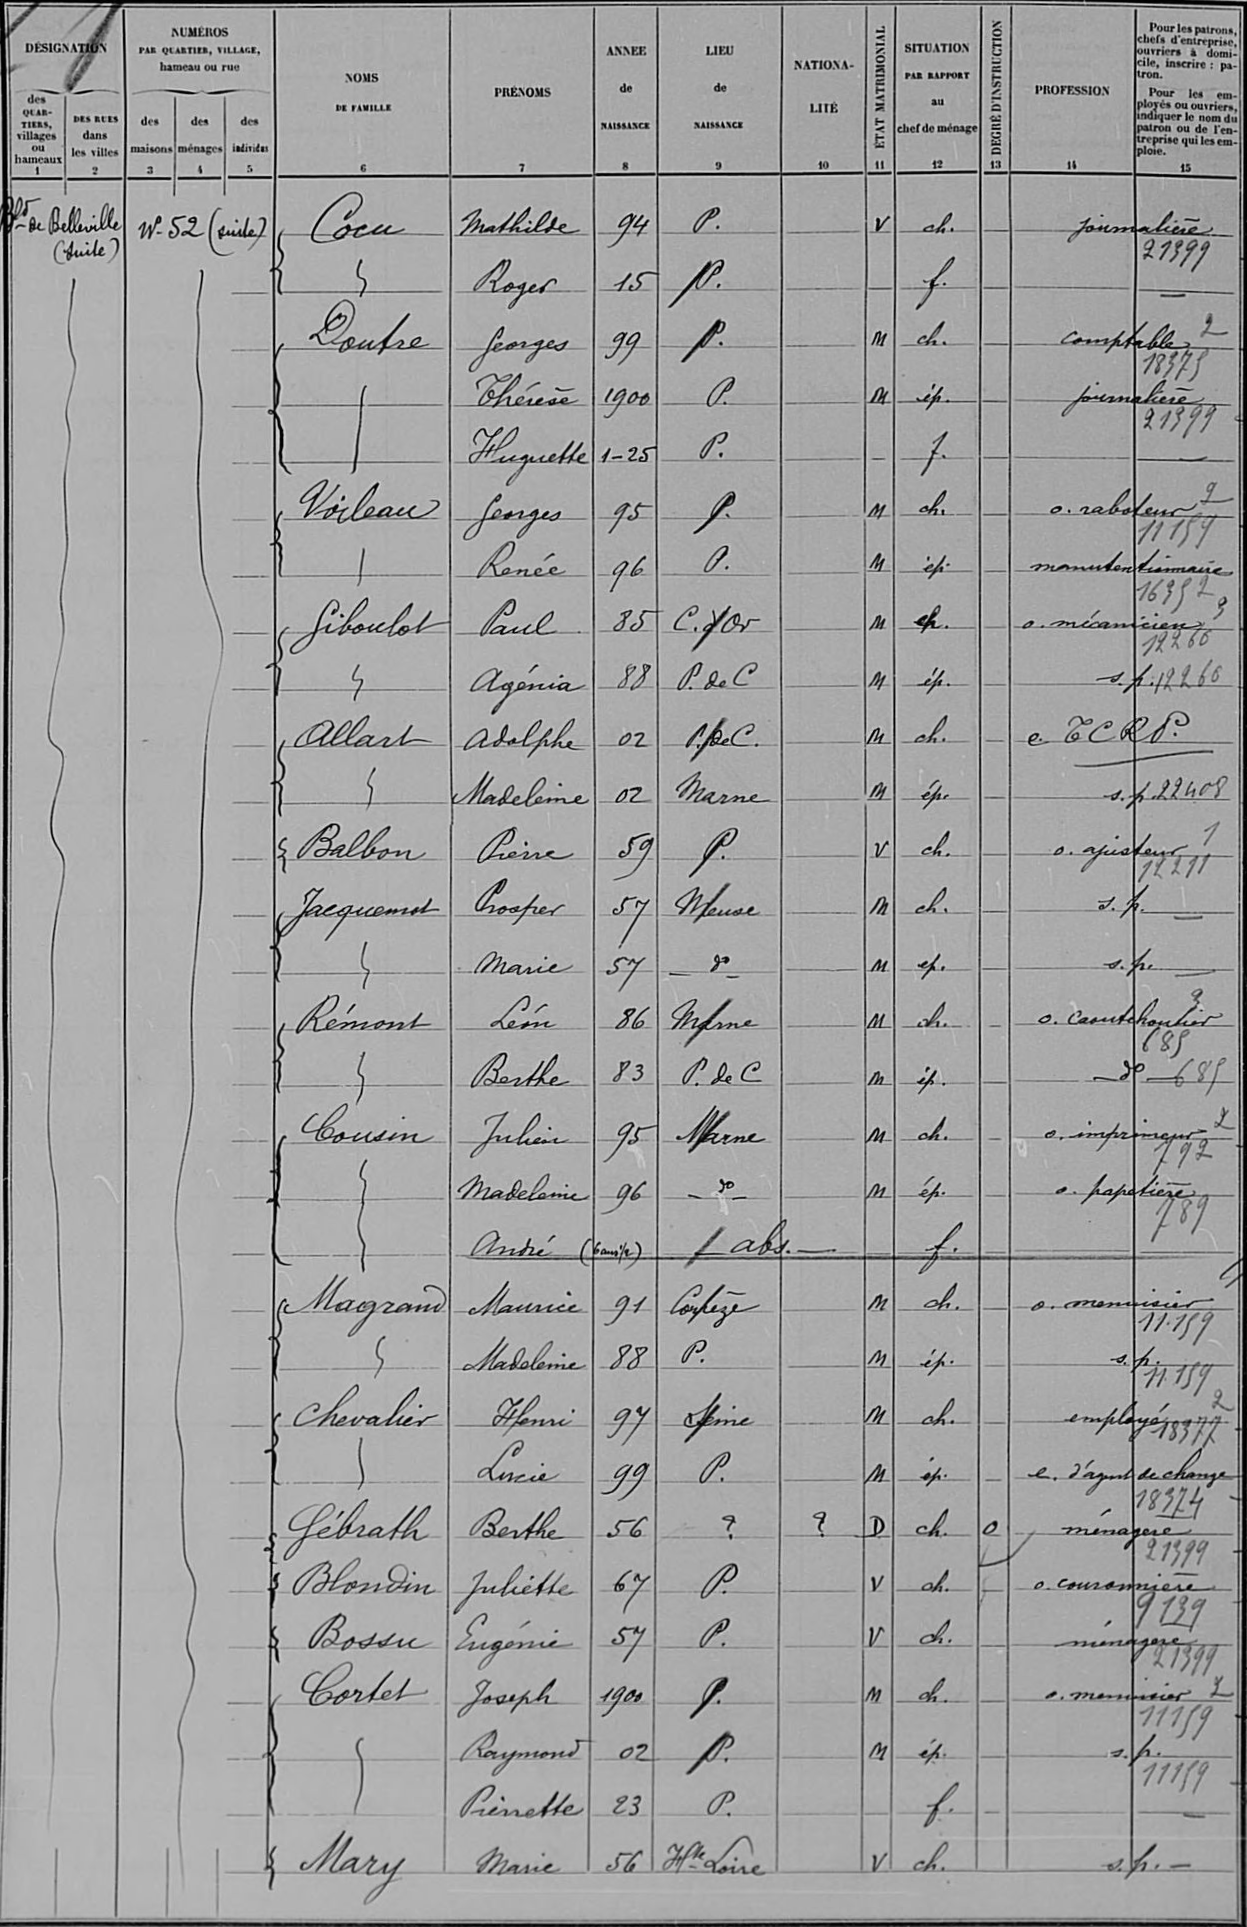Cocu/Mathilde/94/P /¤/V/ch /¤/journalière/¤
¤/Roger/15/P /¤/¤/f /¤/¤/¤/¤
Doutre/Georges/99/P /¤/M/ch /¤/comptable/¤
¤/Thérèse/1900/P /¤/M/ép /journalière/¤
¤/Huguette/1 25/P /¤/¤/f /¤/¤/¤/¤
Voileau/Georges/95/P /¤/M/ch /¤/o raboteur/¤
¤/Renée/96/P /¤/M/ép /¤/manutentionnaire/¤
Giboulot/Paul/85/C d'Or/¤/M/ep /¤/o mécanicien/¤
¤/Agénia/88/P de C/¤/M/ép /¤/s p /12260
Allart/Adolphe/02/P de C /¤/M/ch /¤/e C CRP /¤/¤
¤/madeleine/02/Marne/¤/M/ép /¤/s p /22408
Balbon/Pierre/59/P /¤/V/ch /¤/o ajusteur/122111
Jacquemot/Prosper/57/Meuse/¤/M/ch /¤/s p /¤/¤
¤/Marie/57/d°/¤/M/ep /¤/s p /¤/¤
Rémont/Léon/86/Marne/¤/M/ch /¤/o caoutchoutier/¤
¤/Berthe/83/P de C/¤/M/ép /¤/d°/685
Cousin/Julien/95/Marne/¤/M/ch /¤/o imprimeur/¤
¤/Madeleine/96/d°/¤/M/ép /¤/o papetière/¤
¤/André/(6 ans 1 2)/abs /¤/¤/f /¤/¤/¤/¤
Magrand/Maurice/91/Corrèze/¤/M/ch /¤/o menuisier/¤
¤/Madeleine/88/P /¤/M/ép /¤/s p /¤
Chevalier/Henri/97/Seine/¤/M/ch /¤/employé/¤
¤/lucie/99/P /¤/M/ép /¤/e d'agent de change/¤
Gébrath/Berthe/56/¤/¤/D/ch /O/ménagere/¤
Blondin/Juliette/67/P /¤/V/ch /¤/o couronnière/¤
Bossu/Eugénie/57/P /¤/V/ch /¤/ménagere/¤
Cortet/Joseph/1900/P /¤/M/ch /¤/o menuisier/¤
¤/Raymond/02/P /¤/M/ép /¤/s p /¤
¤/Pierrette/23/P /¤/¤/f /¤/¤/¤/¤
Mary/Marie/56/Hte Loire/¤/V/ch /¤/s p /¤/¤
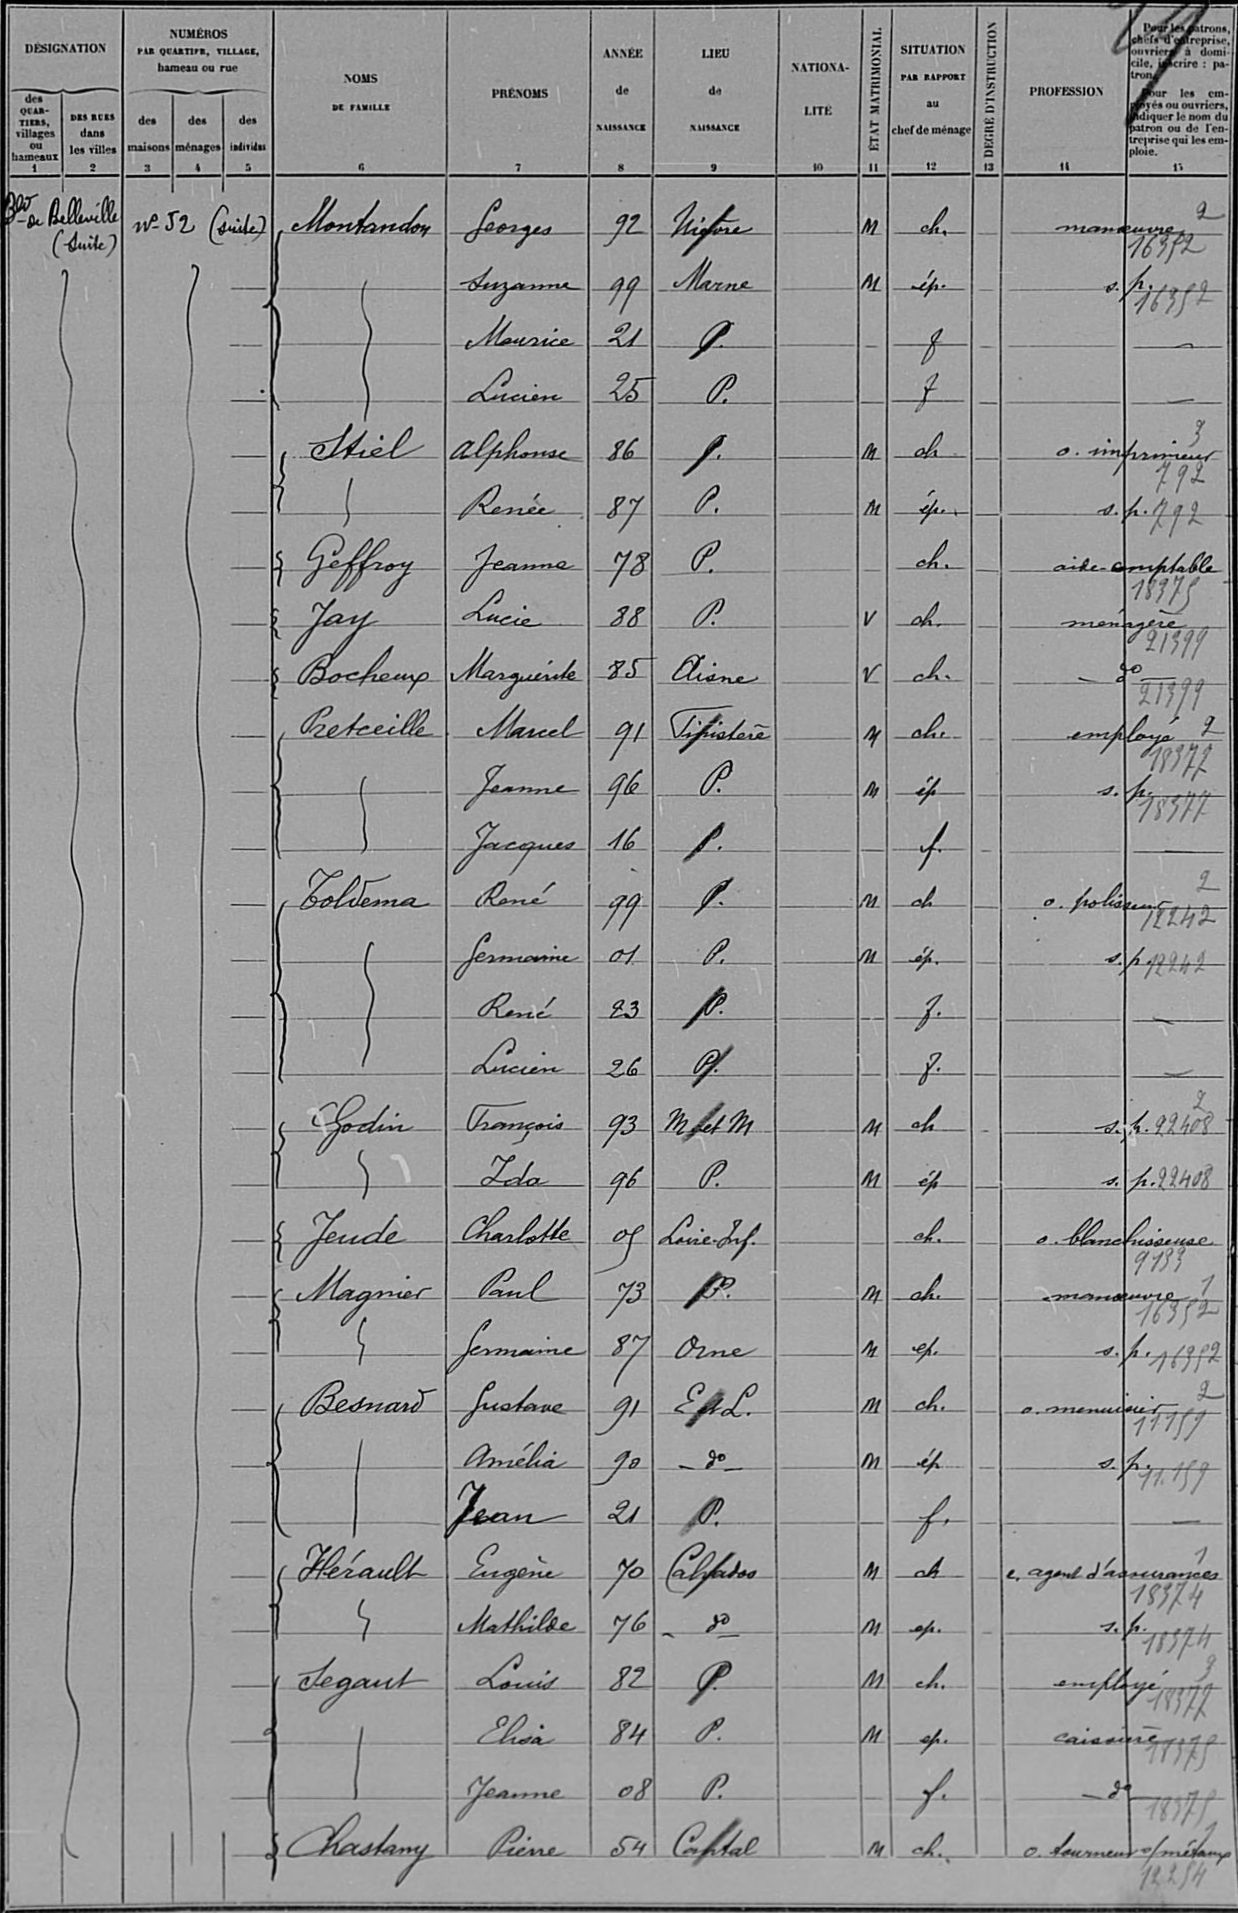Montandon/Georges/92/Nièvre/¤/M/ch /¤/manoeuvre/¤
¤/Suzanne/99/Marne/¤/M/ép /¤/s p /¤
¤/Maurice/21/P /¤/¤/f/¤/¤/¤/¤
¤/Lucien/25/P /¤/¤/f/¤/¤/¤/¤
Shiel/Alphonse/86/P /¤/M/ch/¤/o imprimeur/¤
¤/Renée/87/P /¤/M/ép /¤/s p /792
Geffroy/Jeanne/78/P /¤/¤/ch /¤/aide-comptable/¤
Jay/Lucie/88/P /¤/V/ch /¤/ménagere/¤
Bocheux/Marguerite/85/Aisne/¤/V/ch /¤/d°/¤/¤
Pretuille/Marcel/91/Finistère/¤/M/ch /¤/employé/¤
¤/Jeanne/96/P /¤/M/ép/¤/s p /¤
¤/Jacques/16/P /¤/¤/f /¤/¤/¤/¤
Boldena/René/99/P /¤/M/ch/¤/o polisseur/¤
¤/Germaine/01/P /¤/M/ép /¤/s p /12242
¤/René/23/P /¤/¤/f /¤/¤/¤/¤
¤/Lucien/26/P /¤/¤/f /¤/¤/¤/¤
Godin/François/93/M et M/¤/M/ch/¤/s p /22408 2
¤/ida/96/P /¤/M/ép/¤/s p /22408
Jeude/Charlotte/05/Loire-Inf/¤/¤/ch /¤/o blanchisseuse/¤
Magnier/Paul/73/P /¤/M/ch /manoeuvre/¤
¤/Germaine/87/Orne/¤/M/ep /¤/s p /16352
Besnard/Gustave/91/E et L /¤/M/ch /¤/o menuisier/¤
¤/Amélia/90/d°/¤/M/ép/¤/s p /¤
¤/Jean/21/P /¤/¤/f /¤/¤/¤/¤
Hérault/Eugène/70/Calvados/¤/M/ch/e agent d'assurances/¤
¤/Mathilde/76/d°/¤/M/ep /¤/s p /18374
Segaut/Louis/82/P /¤/M/ch /¤/employé/¤
¤/Elisa/84/P /¤/M/ep /¤/caissière/¤
¤/Jeanne/08/P /¤/¤/f /¤/d°/18375
Chastamy/Pierre/54/Cantal/¤/M/ch /¤/o tourneur s- métaux/¤
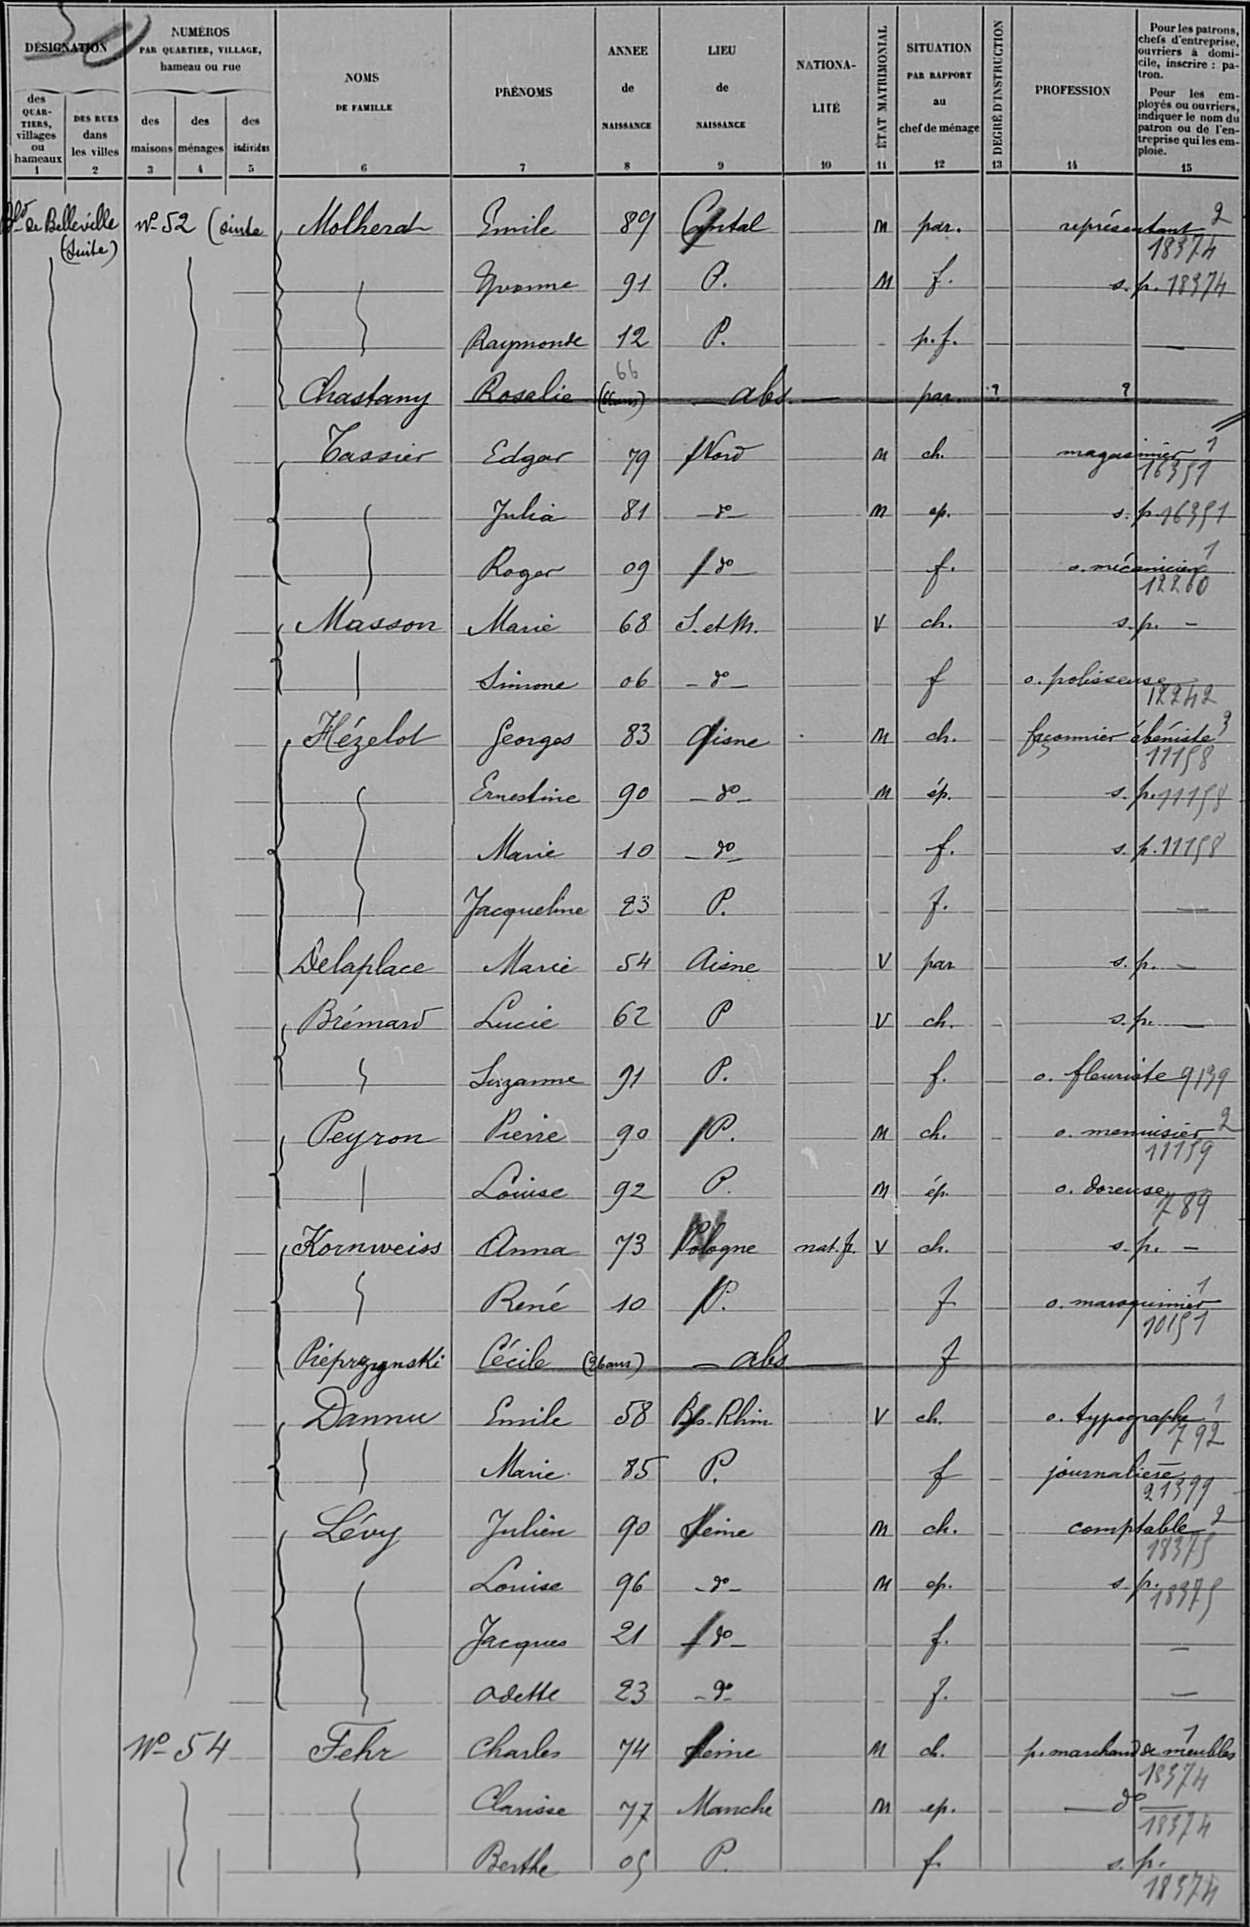Molherat/Emile/89/Cantal/¤/M/par /¤/représentant/¤
¤/Yvonne/91/P /¤/M/f /¤/s p /18374
¤/Raymonde/12/P /¤/¤/p f /¤/¤/¤/¤
Chastany/Roselie/(66 ans) 66/abs/¤/¤/par/¤/¤/¤/¤
Cassier/Edgar/79/Nord/¤/M/ch /¤/magasinier/¤
¤/Julia/81/d°/¤/M/ep /¤/s p/16351
¤/Roger/09/d°/¤/¤/f /¤/o mécanicien/12260 1
Masson/Marie/68/S et M /¤/V/ch /¤/s p /¤/¤
¤/Simone/06/d°/¤/¤/f/¤/o polisseuse/¤
Hézelot/Georges/83/Aisne/¤/M/ch /¤/façonnier ébéniste/¤
¤/ernestine/90/d°/¤/M/ép /¤/s p /11158
¤/Marie/10/d°/¤/¤/f /¤/s p /11158
¤/jacqueline/23/P /¤/¤/f /¤/¤/¤/¤
Delaplace/Marie/54/Aisne/¤/V/par/¤/s p /¤/¤
Brémard/Lucie/62/P/¤/V/ch /¤/s p /¤/¤
¤/Suzanne/91/P /¤/¤/f /¤/o fleuriste/9139
Peyron/Pierre/90/P /¤/M/ch /¤/o menuisier/¤
¤/Louise/92/P /¤/M/ép /¤/o doreuse/¤
Kornweiss/Anna/73/Pologne/nat fr /V/ch /¤/s p /¤/¤
¤/rené/10/P /¤/¤/f/¤/o maroquinier/¤
Préprzynski/Cécile/(26 ans)/abs/¤/¤/f/¤/¤/¤/¤
Dannu/Emile/58/Bs Rhin/¤/V/ch /¤/o typographe/¤
¤/Marie/85/P /¤/¤/f/¤/journaliere/¤
Lévy/Julien/90/Seine/¤/M/ch /¤/comptable/¤
¤/louise/96/d°/¤/M/ep /¤/s p /18375
¤/Jacques/21/d°/¤/¤/f /¤/¤/¤/¤
¤/Odette/23/d°/¤/¤/f /¤/¤/¤/¤
Fehr/Charles/74/Seine/M/ch /¤/p marchand de meubles/¤
¤/Clarisse/77/Manche/¤/M/ep /¤/d°/¤
¤/Berthe/05/P /¤/¤/f/¤/s p /¤